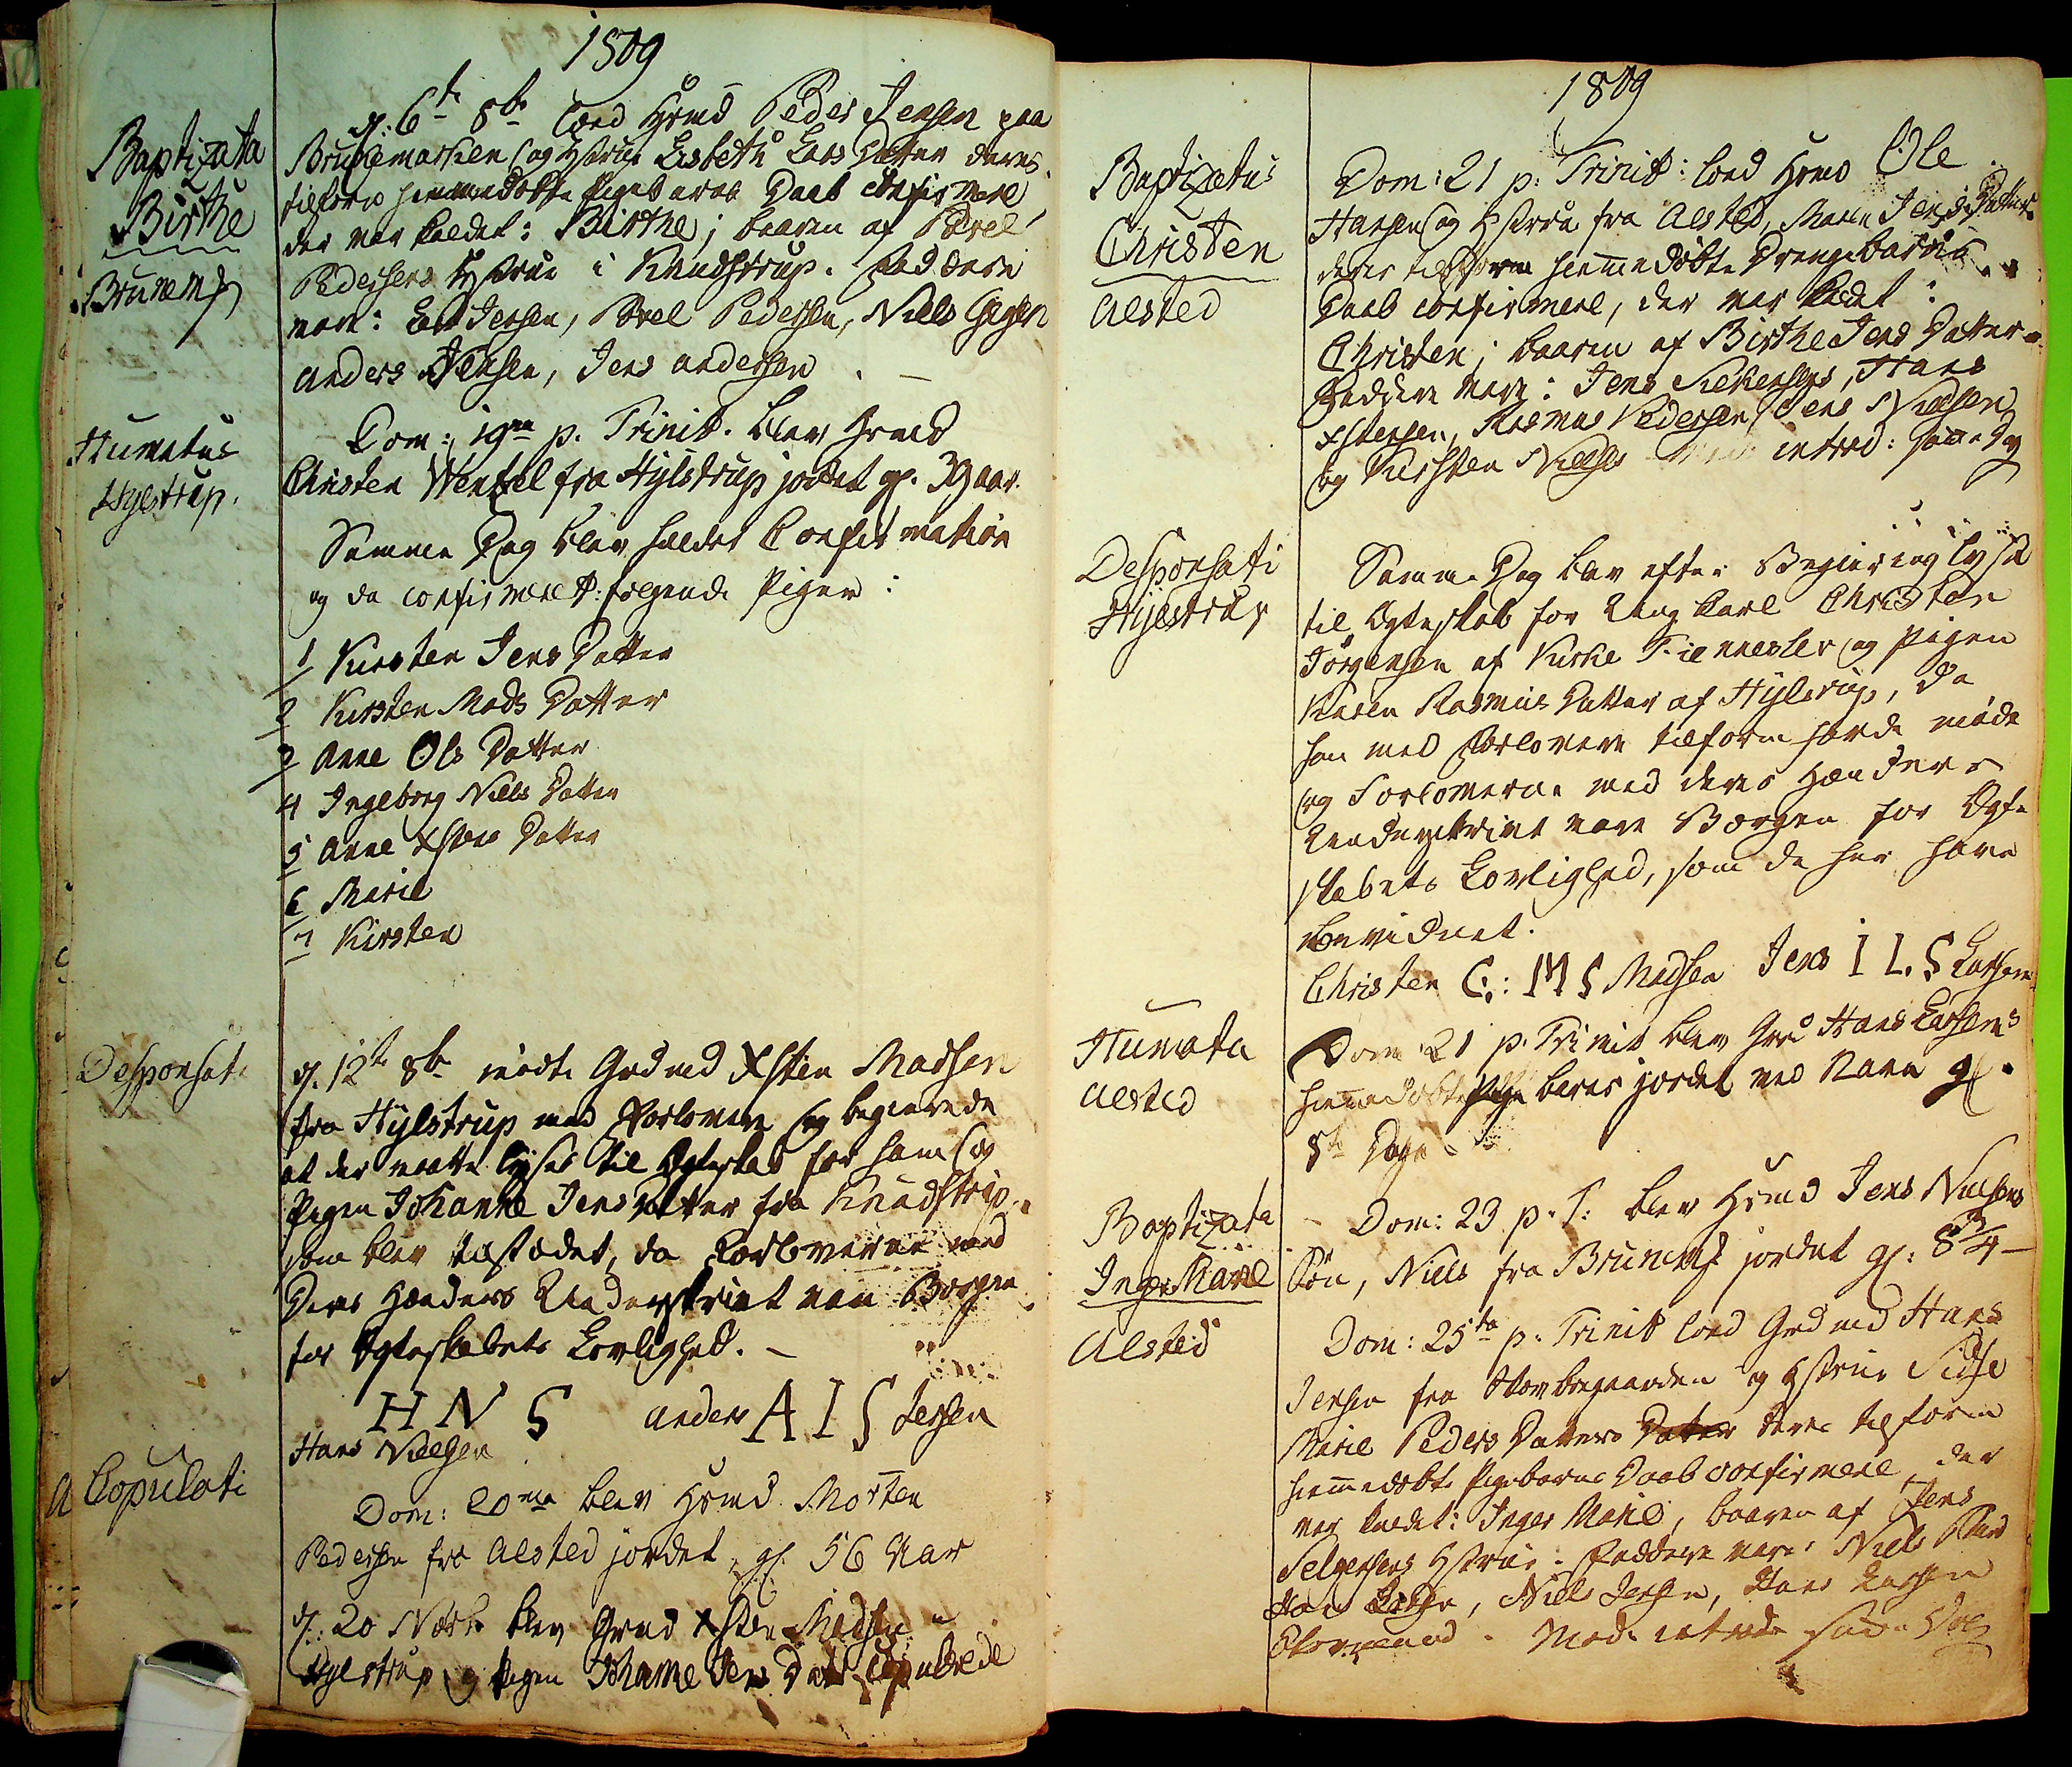Baptizata
Birthe
Brunem##
Humatus
Hylstrup
Desponsati
Copulati
1809
d. 6te 8br loed Hsmd Peder Jensen paa
Brunemarken og Hstrue Lisbeth Lars Datter deres
tilforn hiemmedøbte Pigebarns Daab confirmere
der var kaldet: Birthe; baaren af Povel
Pedersens Hstrue i Knudstrup: Faddere
vare: Lars Jensen, Povel Pedersen, Niels Glg##
Anders Jensen, Jens Andersen
Dom: 19na p. Trinit. blev Hsmd
Christen Wentzel fra Hylstrup jordet gl: 39 Aar.
Samme Dag blev holdet Confirmation
og da confirmeret: følgende Piger:
1 Kirsten Jens Datter
2 Kirsten Mads Datter
3 Anne Ols Datter
4 Ingeborg Niels Datter
5 Anne Xstens Datter
6 Marie
7 Kirsten
d. 12te 8b mødte Grdmd Xsten Madsen
fra Hylstrup med Forlovere og begierede
at der maatte lyses til Ægteskab for ham og
Pigen Johanne Jens Datter fra Knudstrup
som blev tilstædet, da Forloverne ved
Deres Hænders Underskrivt var Borgen
for Ægteskabets Lovelighed.
N N S
Hans Nielsen
Anders A I S  Jessen
Dom: 20ma blev Hsmd Morten
Pedersen fra Alsted jordet gl 56 Aar
d: 20 Novbr blev Xsten Madsen i
Hylstrup og Pigen Johanne Jens Dat## copulerede
Baptizatus
Christen
Alsted
Desponsati
Hylstrup
Humata
Alsted
Baptizata
Inger Marie
Alsted
1809
Dom: 21 p. Trinit: loed Hsmd Ole
Hansen og Hstrue fra Alsted, Maren Jens Datter
deres tilforn hiemmedøbt Drengebarns
Daab confirmere, der var kaldet:
Christen; baaren af Birthe Jens Datter.
Faddere vare: Jens Silkensen, Hans
Xstensen, Rasmus Pedersen, Jens Nielsen
og Kirsten Nielses Mod: introd: samme Dag
Samme Dag blev efter Begiering lyst
til Ægteskab for Ung Karl Christen
Jørgensen af Kirke Fienneslev og Pigen
Karen Rasmus Datter af Hylstrup, da
han med Forlovere tilforn havde mødt
og Forloverne med derrs Hænders
Underskrivt vare Borgen for Ægte
skabets Lovligged, som de har have
bevidnet.
Christen C: M S Madsen Jens I L S Larsen
Dom 21 p: Trinit blev Grd Hans Larsens
hiemedøbte Pige barn jordet ved navn gl.
5te Dage
Dom: 23 p: T: blev Hsmd Jens Nielsens
Søn, Niels fra Brunem## jordet gl: 8¾
Dom: 25ta p: Trinit loed Grdmd Hans
Jensen fra Skovrboegaarden og Hstrue Sidse
Marie Peders Datters Dater deres tilforn
hiemmedøbt Pigebarns Daab confirmere, der
var kaldet: Inger Marie, baaren af Jens
Selgersens Hstrue. Faddere vare Niels ##
H## Lerhe, Niels Jensen, Hans Larsen
Skovgaard. Mod: introd: samme Dag
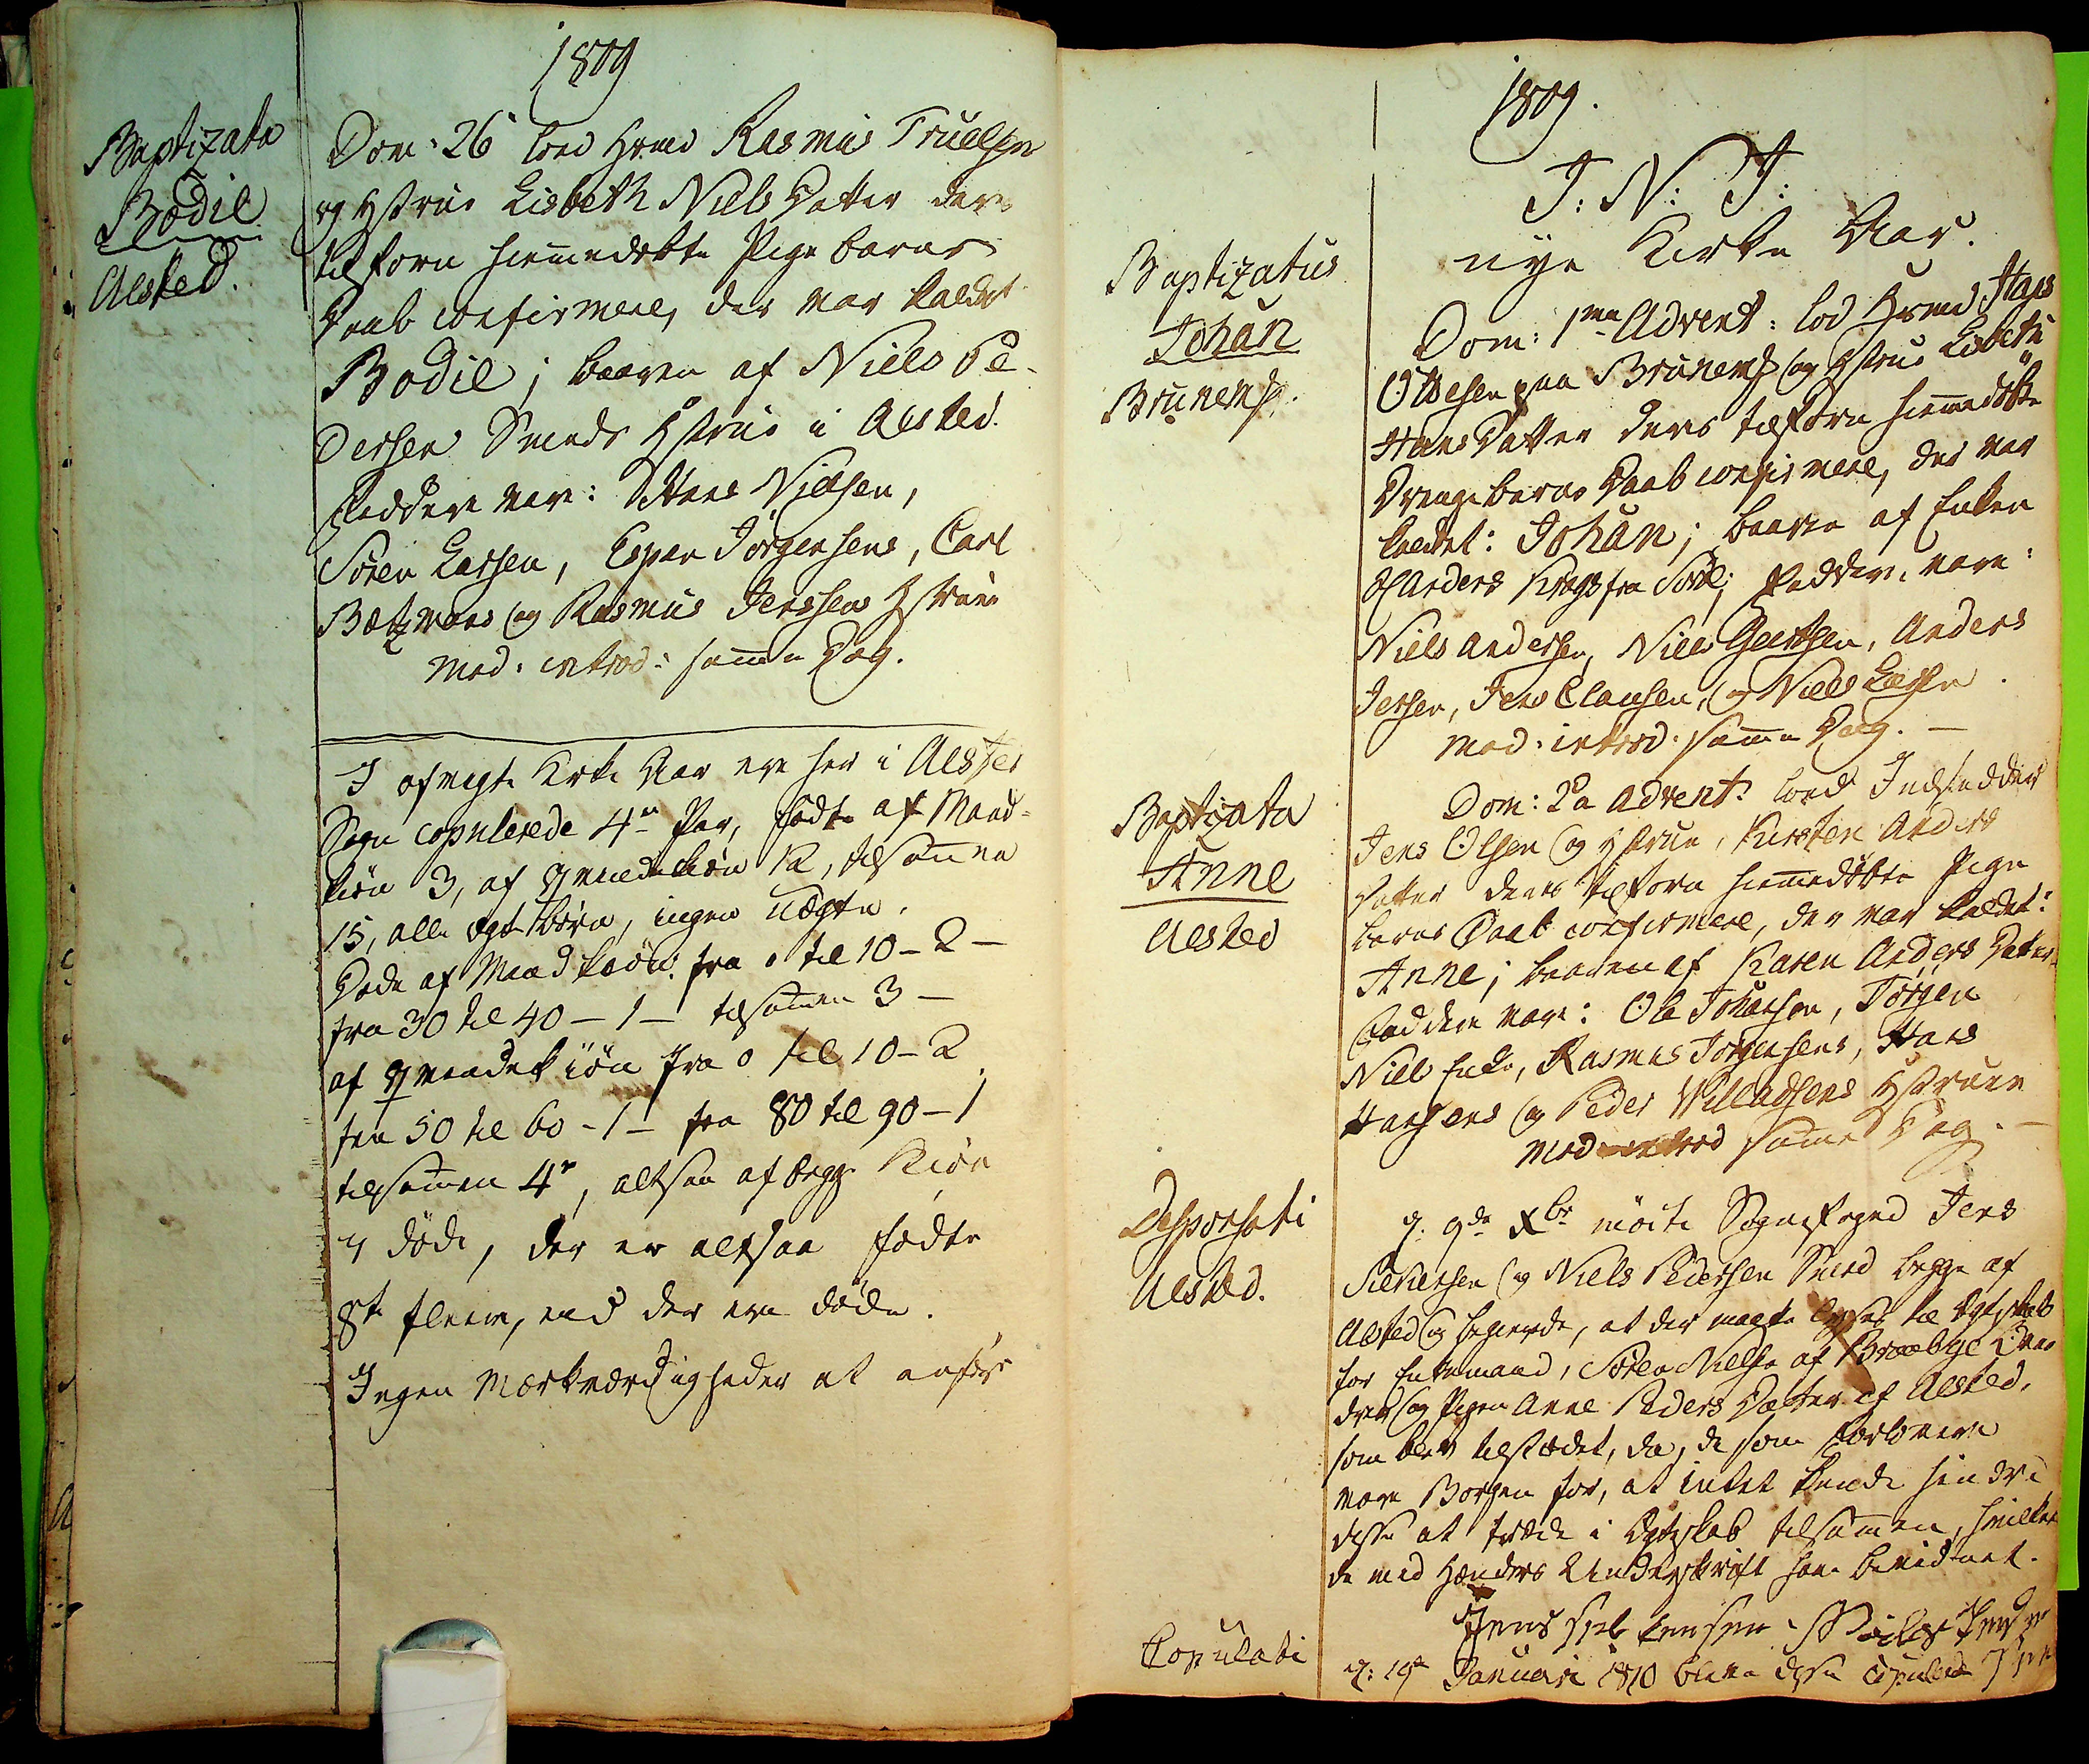Baptzata
Bodil
Alsted.
1809
Dom 26 loed Jsmd Rasmus Truelsen
og Hstrue Lisbeth Niels Datter deres
tilforn hiemmedøbte Pigebarns
Daab confirmere, der var kaldet
Bodil, baaren af Niels Pe¬
dersen Smeds Hstrue i Alsted.
Faddere var: Hans Nielsen,
Søren Larsen, Espen Jørgensens, Carl
Bætzmans og Rasmus Jenssens Hstruer
Mod: introd: samme Dag.
I afvigte Krke Aar ere her i Alsted
Sogn copulerede 4re Par, fødte af Mand¬
kiøn 3, af Qvindekiøn 12, tilsammen
15, alle Ægtebørn, ingen uægte.
Dode af Mandkiøn fra . til 10 - 2 -
fra 30 til 40 - 1 - tilsammen 3 -
af Qvindekiøn fra 0 til 10 - 2
ten 50 til 60 - 1 - fra 80 til 90 - 1
til sammen 4## altsaa af begge Kiøn
7 døde, der er altsaa fødte
8te flere end der ere døde
Inge Mærkværdigheder at anføre
Baptizatus
Johan
Brunem##.
Baptizata
Anne
Alsted
Desponsati
Alsted.
Copulati
1809.
J: N: J:
nye Kirke Aar.
Dom: 1ma Advent: lod Hsmd Hans
Ottesen paa Brune## og Hstrue Lisbeth
Hans Datter deres tilforn hiemmedøbte
Drenhebarns Daab confirmere, der var
kaldet: Johan; baaren af Enken
Sl Anders Krogs fra Sorøe; Faddere vare:
Niels Anderssen, Niels Geertsen, Anders
Jensen, Jens Clausen, og Niels Larsen
Mod: introd: samme Dag.
Dom: 2## a Advent. loed Indsidder
Jens Olsen og Hstrue Kirsten Anders
Datter deres tilforn hiemmedøbte Pige
barns Daab confirmere, der var kaldet:
Anne; baaren af Karen Anders Datter
Faddere vare: Ole Johansen, Jørgen
Niels Enke, Rasmus Jørgensens, Hans
Hansens og Peder Willadsens Hstruer.
Mod introd samme Dag
d: 9de Xbr mødte Sognefoged Jens
Silkensen og Niels Pedersen Smed, begge af
Alsted og begierede, at der maatte lyses til Ægteskab
for Enkemand, Søren Nielsen aff Braabye, Over
drev og Pigen Anne Peders Datter af Alsted,
som blev tilstædet, da de som Forlovere
vare Borgen for, at intet kunde hindre
disse at træde i Ægteskab tilsammen, hvilket
de med Hænders Underskrivt have bevidnet.
Jens Sikelsen Niels Pedersen
d: 19de Januari 1810 blef disse copulerede